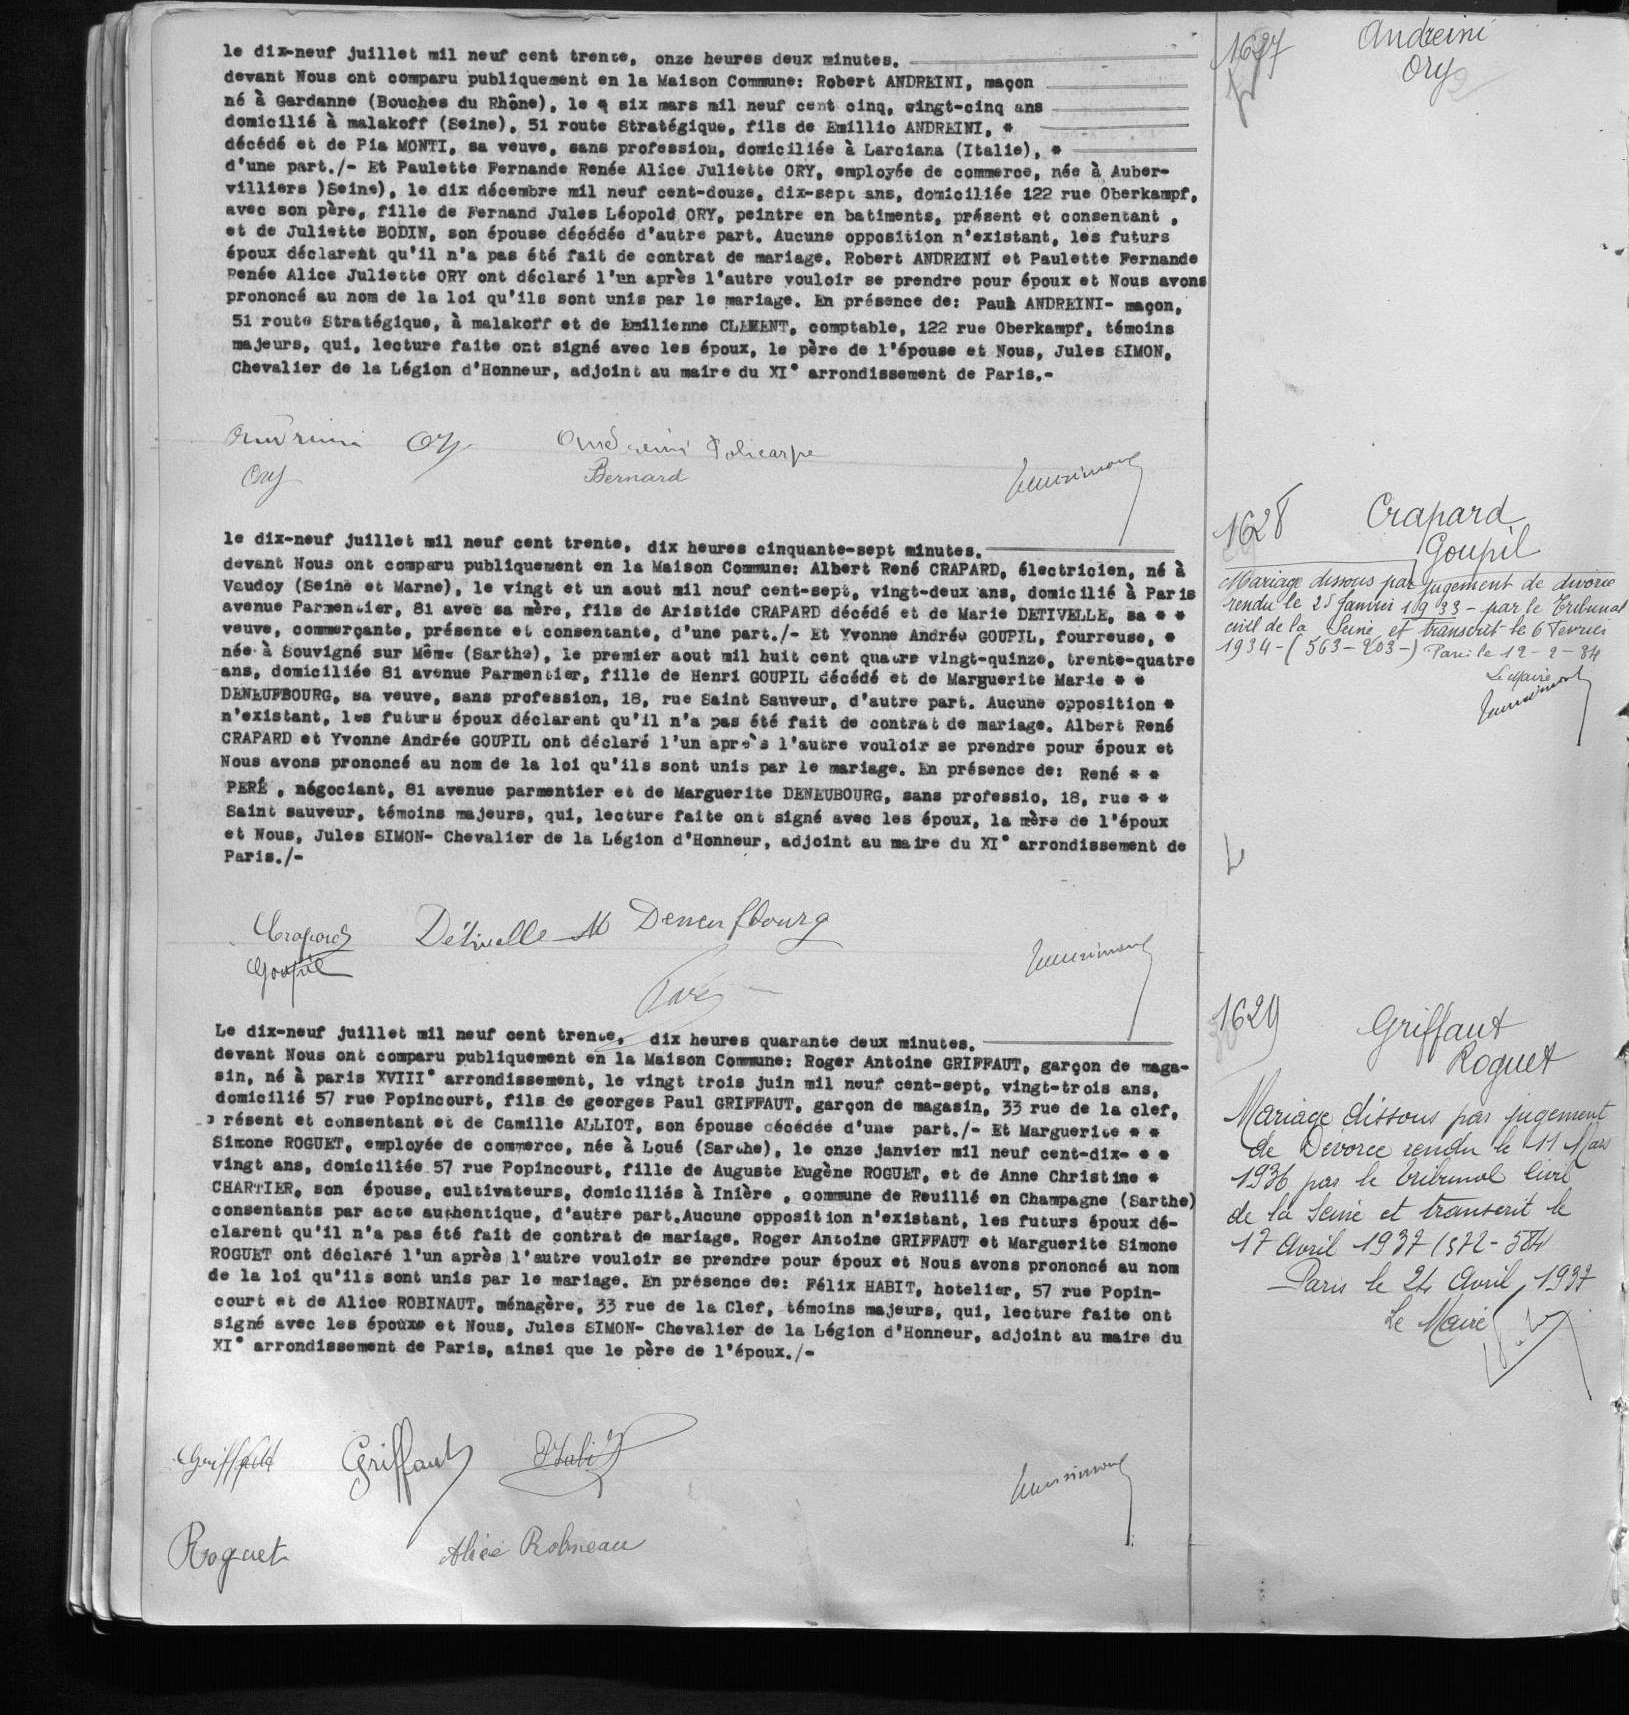le dix-neuf juillet mil neuf cent trente, onze heures deux minutes.-
devant Nous ont comparu publiquement en la Maison Commune: Robert ANDERINI, maçon -
né à Gardanne (Bouches du Rhône), le * six mars mil neuf cent cinq, vingt-cinq ans -
domicilié à malakoff (Seine), 51 route Stratégique, fils de Emillio ANDREINI, *-
décédé et de Pia MONTI, sa veuve, sans profession, domiciliée à Larciana (Italie), * -
d'une part ./-Et Paulette Fernande Renée Alice Juliette ORY, employée de commerce, née à Auber-
villiers) Seine), le dix décembre mil neuf cent-douze, dix-sept ans, domiciliée 122 rue Oberkampf,
avec son père, fille de Fernand Jules Léopold ORY, peintre en batiments, présents et consentant,
et de Juliette BODIN, son épouse décédée d'autre part. Aucune oppositon n'existant, les futurs
époux déclarent qu'il n'a pas été fait de contrat de mariage. Robert ANDREINI et Paulette Fernande
Renée Alice Juliette ORY ont déclaré l'un après l'autre vouloir se prendre pour époux et Nous avons
prononcé au nom de la loi qu'ils sont unis par le mariage. En présence de: Paul ANDREINI-maçon,
51 route Stratégique, à malakoff et de Emilienne CLEMENT, comptable, 122 rue Oberkamf, témoins
majeurs, qui, lecture faite ont signé avec les époux, le père de l'épouse et Nous, Jules SIMON,
Chevalier de la Légion d'Honneur, adjoint au maire du XI ° arrondissement de Paris.-
le dix-neuf juillet mil neuf cent trente, dix heures cinquante-sept minutes.-
devant Nous ont comparu publiquement en la Maison Commune: Albert René CRAPARD, électricien, né à
Vaudoy (Seine et Marne), le vingt et un aout mil neuf cent-sept, vingt-deux ans, domicilié à Paris
avenue Parmentier, 81 avec sa mère, fils de Aristide CRAPARD décédé et de Marie DETIVELLE, sa * *
veuve, commerçante, présente et consentante, d'une part ./-Et Yvonne André GOUPIL, fourreuse, *
née à Souvigné sur Même (Sarthe), le premier aout mil huit cent quatre vingt-quinze, trente-quartre
ans, domiciliée 82 avenue Parmentier, fille de Henri GOUPIL décédé et de Marguerite Marie * *
DENEUFBOURG, sa veuve, sans profession, 18, rue Saint Sauveur, d'autre part. Aucune opposition *
n'existant, les futurs époux déclarent qu'il n'a pas été fait de contrat de mariage. Albert René
CRAPARD et Yvonne Andrée GOUPIL ont déclaré l'un après l'autre vouloir se prendre pour époux et
Nous avons prononcé au nom de la loi qu'ils sont unis par le mariage. En présence de: René * *
PERE, négociant, 81 avenue parmentier et de Marguerite DENEUBOURG, sans professio, 18, rue * *
Saint sauveur, témoins majeurs, qui, lecture faite ont signé avec les époux, la mère de l'époux
et Nous, Jules SIMON-Chevalier de la Légion d'Honneur, adjoint au maire du XI ° arrondissement de
Paris ./-
Le dix-neuf juillet mil neuf cent trente, dix heures quarante deux minutes.-
devant Nous ont comparu publiquement en la Maison Commune: Roger Antoine GRIFFAUT, garçon de maga-
sin, né à paris XVIII ° arrondissement, le vingt trois juin mil neuf cent-sept, vingt-trois ans,
domicilié 57 rue Popincourt, fils de georges Paul GRIFFAUT, garçon de magasin, 33 rue de la clef,
présent et consentant et de Camille ALLIOT, son épouse décédée d'une part ./-Et Marguerite * *
Simone ROGUET, employée de commerce, née à Loué (Sarthe), le onze janvier mil neuf cent-dix-* *
vingt ans, domiciliée 57 rue Popincourt, fille de Auguste Eugène ROGUET, et de Anne Christine *
CHARTIER, son épouse, cultivateurs, domiciliés à Inière, commune de Reuillé en Champagne (Sarthe)
consentants par acte authentique, d'autre part. Aucune opposition n'existant, les futurs époux dé-
clarent qu'il n'a pas été fait de contrat de mariage. Roger Antoine GRIFFAUT et Marguerite Simone
ROGUET ont déclaré l'un après l'autre vouloir se prendre pour époux et Nous avons prononcé au nom
de la loi qu'ils sont unis par le mariage. En présence de: Félix HABIT, hotelier, 57 rue Popin-
court et de Alice ROBINAUT, ménagère, 33 rue de la Clef, témoins majeurs, qui, lecture faite ont
signé avec les époux et Nous, Jules SIMON-Chevalier de la Légion d'Honneur, adjoint au maire du
XI ° arrondissement de Paris, ainsi que le père de l'époux ./-
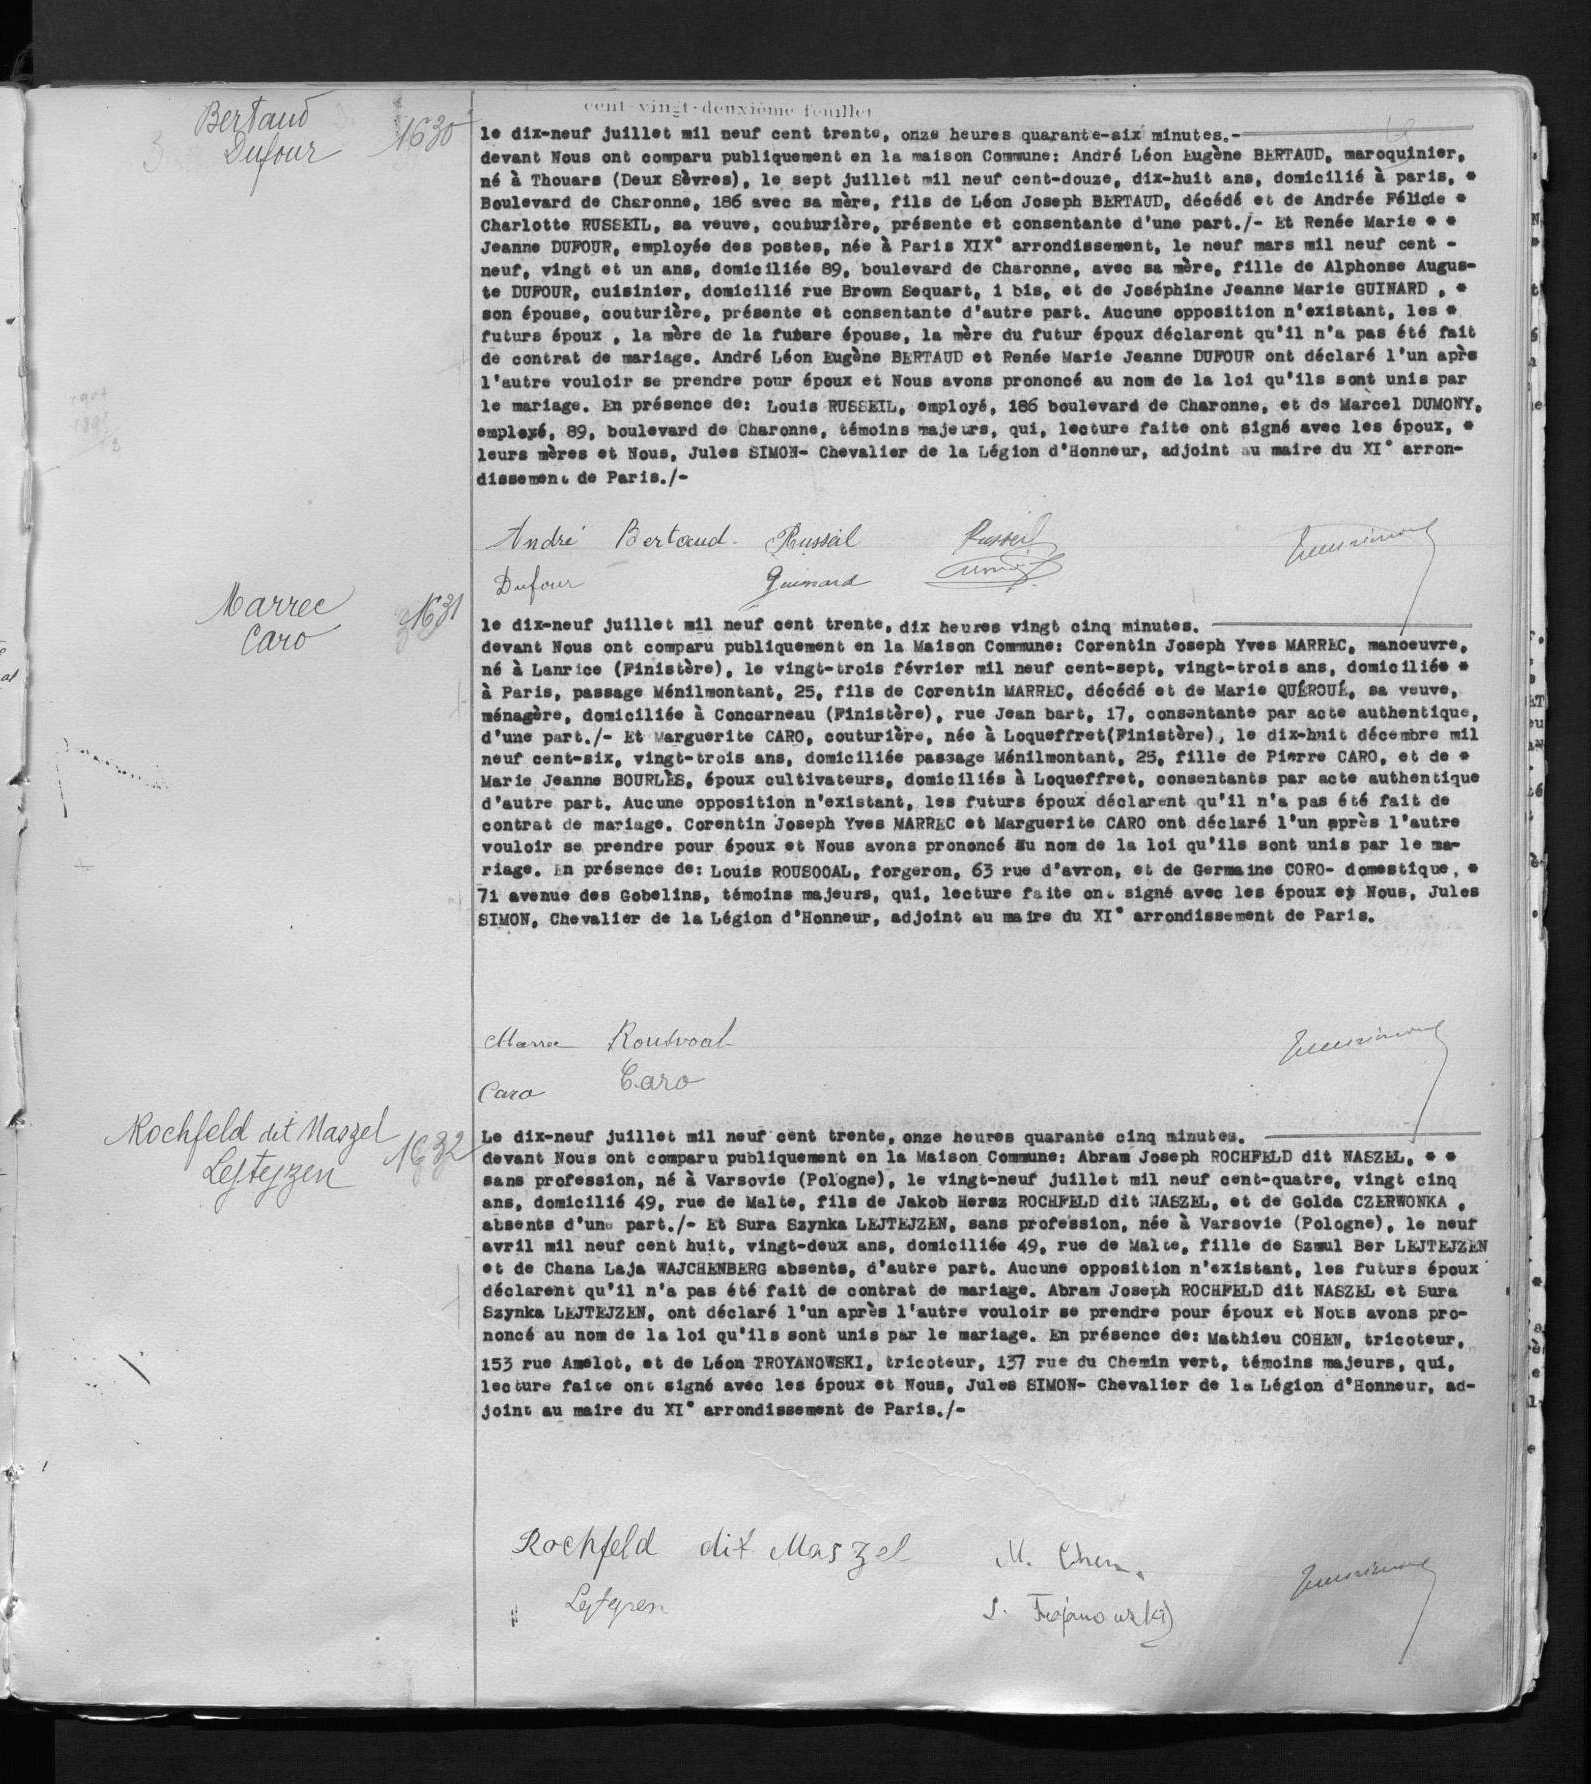le dix-neuf juillet mil neuf cent trente, onze heures quarante-six minutes.-
devant Nous ont comparu publiquement en la maison Commune: André Léon Eugène BERTAUD, maroquinier,
né à Thouars (Deux Sèvres), le sept juillet mil neuf cent-douze, dix-huit ans, domicilié à paris, *
Boulevard de Charonne, 186 avec sa mère, fils de Léon Joseph BERTAUD, décédé et de André Félicie *
Charlotte RUSSEIL, sa veuve, couturière, présente et consentante d'une part ./-Et Renée Marie * *
Jeanne DUFOUR, employée des postes, née à Paris XIX ° arrondissement, le neuf mars mil neuf cent-
neuf, vingt et un ans, domiciliée 89, boulevard de Charonne, avec sa mère, fille de Alphonse Augus-
te DUFOUR, cuisinier, domiciliée rue Brown Sequart, 1 bis, et de Joséphine Jeanne Marie GUINARD, *
son épouse, couturière, présente et consentante d'autre part. Aucune opposition n'existant, les *
futurs époux, la mère de la future épouse, la mère du futur époux déclarent qu'il n'a pas été fait
de contrat de mariage. André Léon Eugène BERTAUD et Renée Marie Jeanne DUFOUR ont déclaré l'un aprs
l'autre vouloir se prendre pour époux et Nous avons prononcé au nom de la loi qu'ils sont unis par
le mariage. En présence de: Louis RUSSEIL, employé, 196 boulevard de Charonne, et de Marcel DUMONY,
employé, 89, boulevard de Charonne, témoins majeurs, qui, lecture faite ont signé avec les époux, *
leurs mères et Nous, Jules SIMON-Chevalier de la Légion d'Honneur, adjoint au maire du XI ° arron-
dissement de Paris ./-
le dix-neuf juillet mil neuf cent trente, dix heures vingt cinq minutes .-
devant Nous ont comparu publiquement en la Maison Commune: Corentin Joseph Yves MARREC, manoeuvre,
né à Lanrice (Finistère), le vingt-trois février mil neuf cent-sept, vingt-trois ans, domicilié * *
à Paris, passage Ménilmontant, 25, fils de Corentin MARREC, décédé et de Marie QUEROUE, sa veuve,
ménagère, domiciliée à Concarneau (Finistère), rue Jean bart, 17, consentante par acte authentique,
d'une part ./-Et Marguerite CARO, couturière, née à Loqueffret (Finistère), le dix-huit décembre mil
neuf cent-six, vingt-trois ans, domiciliée passage Ménilmontant, 25, fille de Pierre CARO, et de *
Marie Jeanne BOURLES, époux cultivateurs, domiciliés à Loqueffret, consentants par acte authentique
d'autre part. Aucune opposition n'existant, les futurs époux déclarent qu'il n'a pas été fait de
contrat de mariage. Corentin Hoseph Yves MARREC et Marguerite CARO ont déclaré l'un après l'autre
vouloir se prendre pour époux et Nous avons prononcé au nom de la loi qu'ils sont unis par le ma-
riage. En présence de: Louis ROUS00AL, forgeron, 63 rue d'avron, et de Germain CORO-domestique, *
71 avenue des Gobelins, témoins majeurs, qui, lecture faite ont signé avec les époux et Nous, Jules
SIMON, Chevalier de la Légion d'Honneur, adjoint au maire du XI ° arrondissement de Paris.
Le dix-neuf juillet mil neuf cent trente, onze heures quarante cinq minutes .-
devant Nous ont comparu publiquement en la Maison Commune: Abram Joseph ROCHFELD dit NASZEL, * *
sans profession, né à Varsovie (Pologne), le vingt-neuf juillet mil neuf cent-quatre, vingt cinq
ans, domicilié 49, rue de Malter, fils de Jakob Heraz ROCHFELD dit HASZEL, et de Golda CZEWONKA,
absents d'une part ./-Et Sura Szynka LEJTEJZEN, sans profession, née à Varsovie (Pologne), le neuf
avril mil neuf cent huit, vingt-deux ans, domiciliée 49, rue de Malte, fille de Szmul Ber LEJTEJZEN
et de Chana Laja WAJCHENBERG absents, d'autre part. Aucune opposition n'existant, les futurs époux
déclarent qu'il n'a pas été fait de contrat de mariage. Abram Joseph ROCHFELD dit NASZEL et Sura
Szynka LEJTEJZEN, ont déclaré l'un après l'autre vouloir se prendre pour époux et Nous avons pro-
noncé au nom de la loi qu'ils sont unis par le mariage. En présence de: Mathieu COHEN, tricoteur,
153 rue Amelot, et de Léon TROYANOWSKI, tricoteur, 137 rue du Chemin vert, témoins majeurs, qui,
lecture faite ont signé avec les époux et Nous, Jules SIMON-Chevalier de la Légion d'Honneur, ad-
joint au maire du XI ° arrondissement de Paris ./-